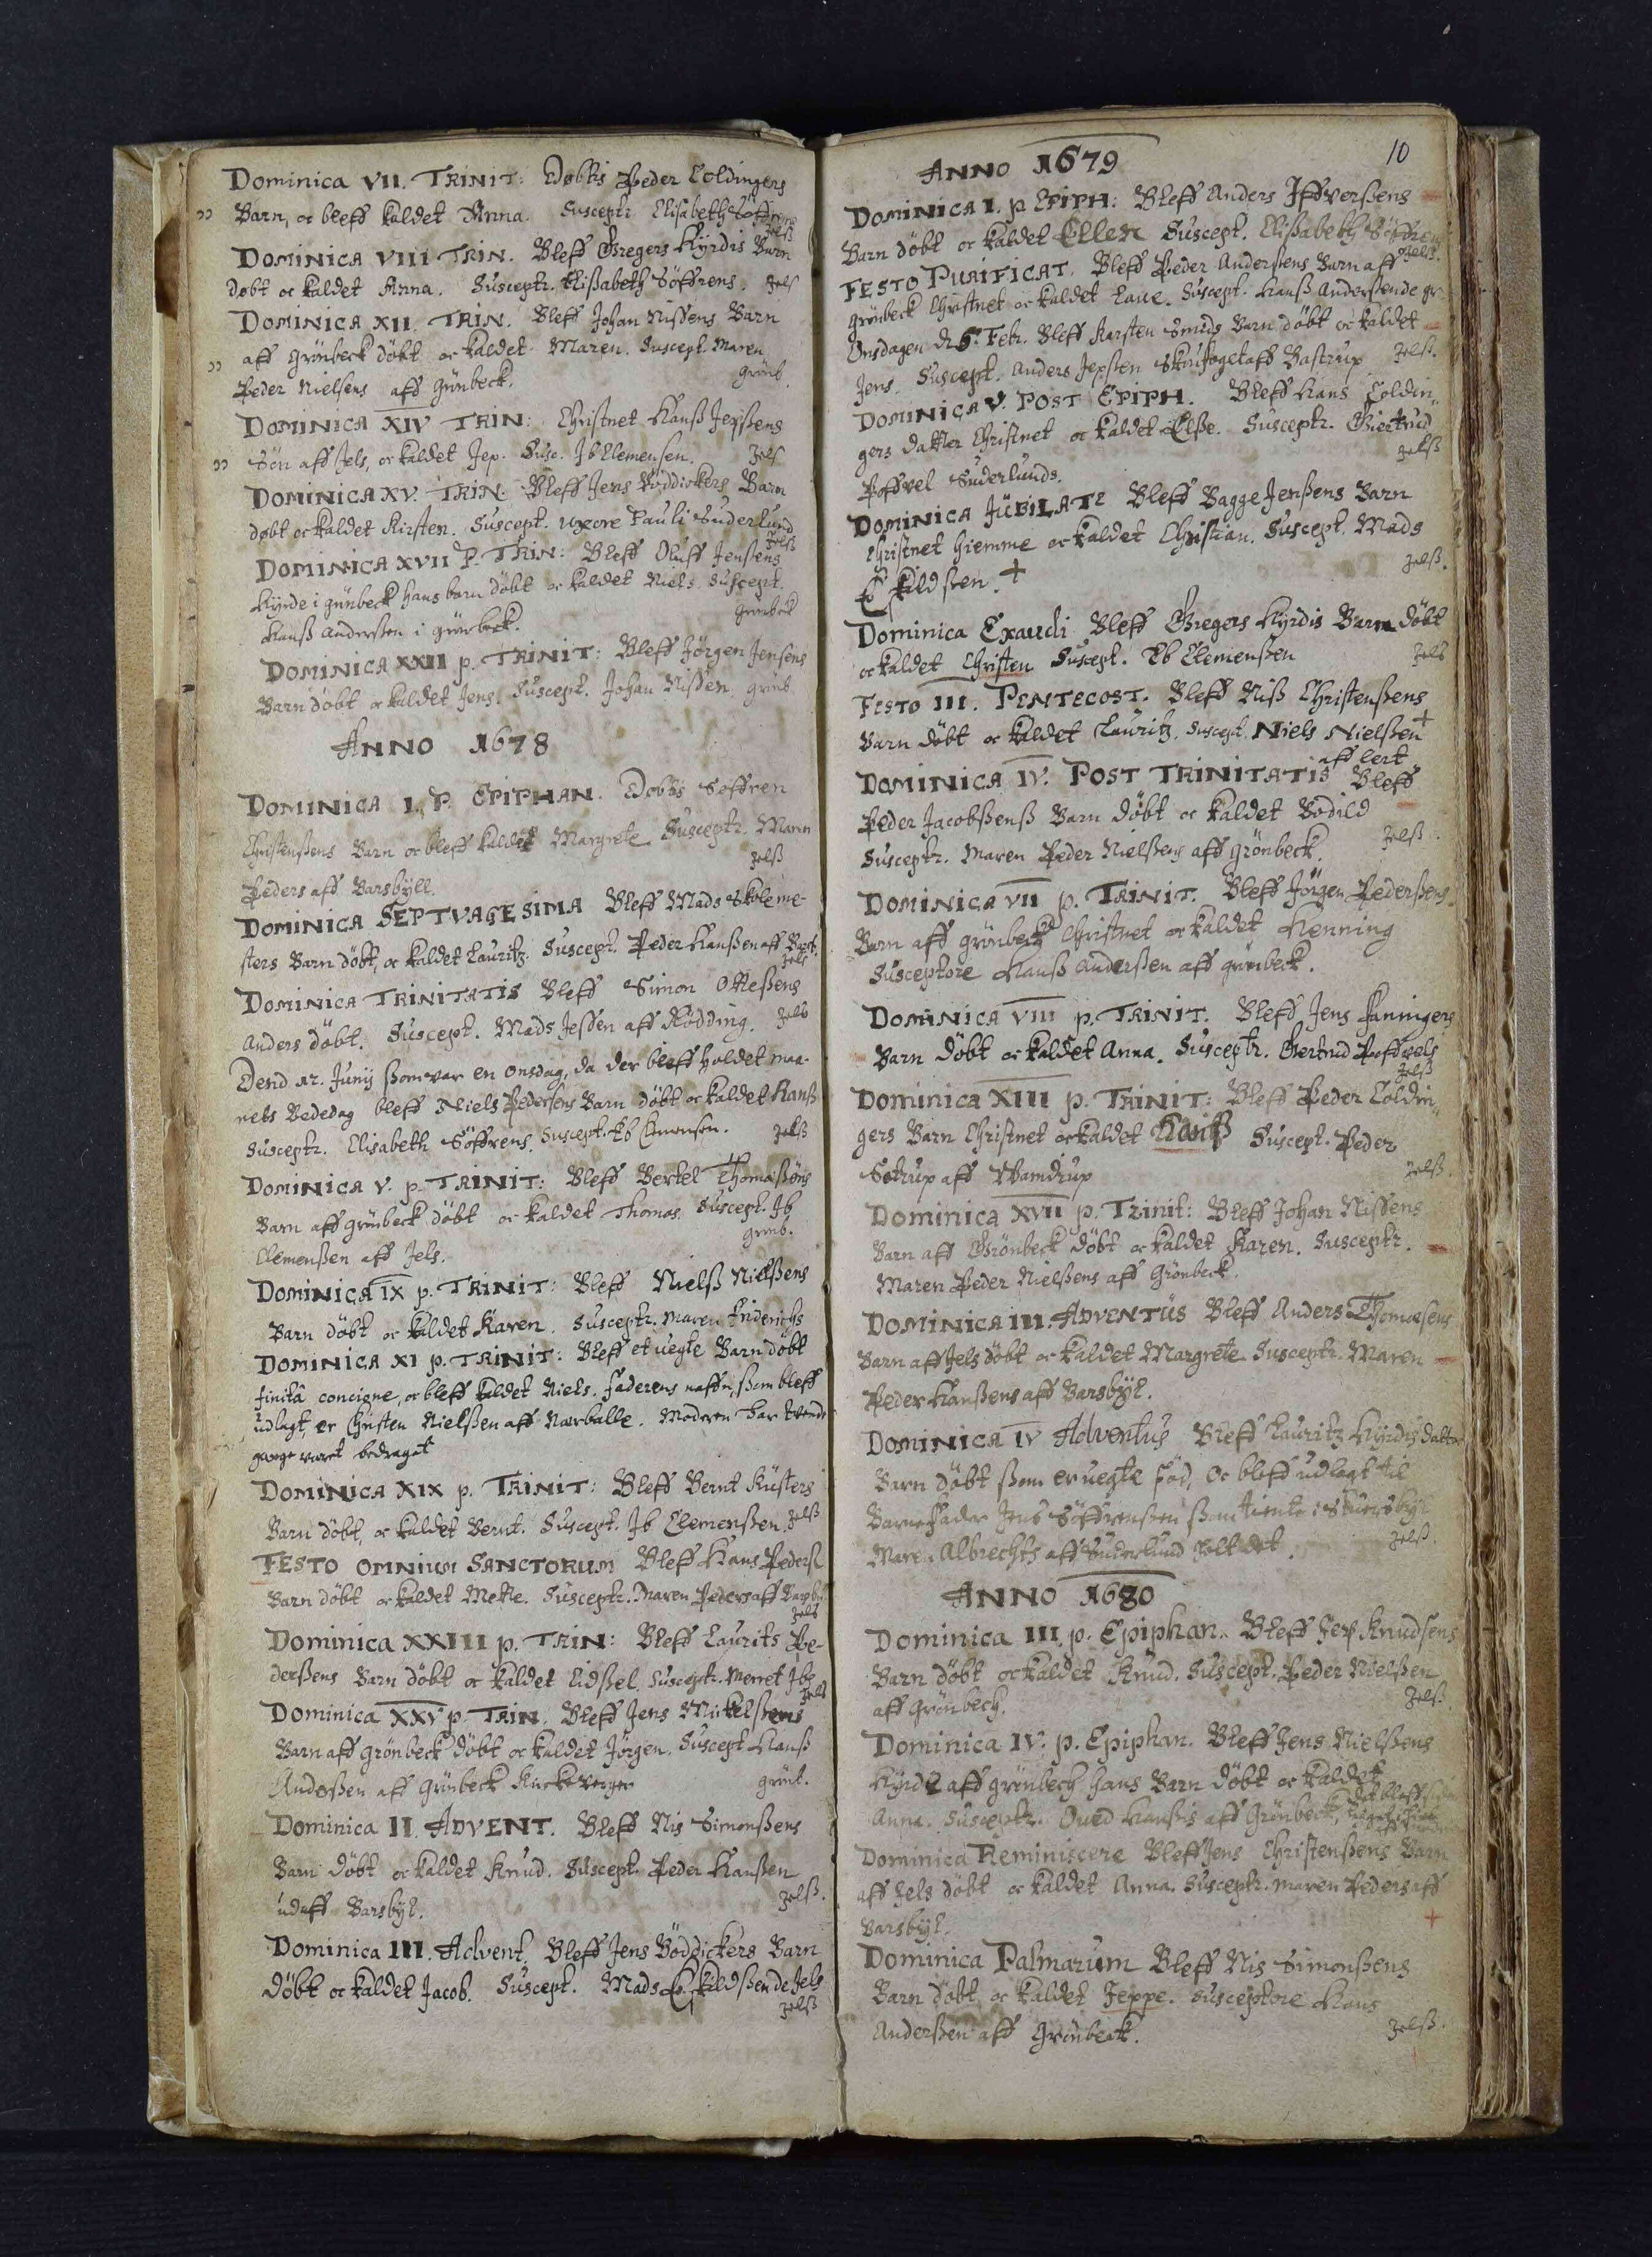Dominica VII TRINIT. Døbtis Peder Coldingers
Barn, oc bleff kaldet Anna. Susceptr Elisabeth Søffre##
Jels
DOMINICA VIII TRIN. Bleff Gregers Hÿrdis Barn
døbt oc kaldet Anna. Susceptr Elisabeth Søffrens. Jels
DOMINICA XII TRIN. Bleff Johan Nissens Barn
aff Grønbeck døbt oc kaldet Maren. Suscept. Maren
Peder Nielsens af Grønbeck.
Grønb
DOMINICA XIV TRIN: Christnet Hans Jepsens
Søn af Jels, oc kaldet Jep. Susc. Ib Clemensen. Jels
DOMINICA XV. TRIN. Bleff Jens Bøddickers Barn
døbt oc kaldet Kirsten. Suscept. Uxore Pauli Suderlund.
Jels
DOMINICA XVII P. TRIN: Bleff Oluf Jensens
Hÿrde i grønbeck hans barn døbt oc kaldet Niels Suscept.
Hans Andersen i grønbeck.
Grønbeck
DOMINICA XXII p. TRINIT: Bleff Jørgen Jensens
Barn døbt oc kaldet Jens. Suscept. Johan Nissen Grønb.
ANNO 1678
DOMINICA I P. EPIPHAN. Døbtis Søffren
Christensens Barn oc bleff kaldet Margrete. Susceptr. Maren
Peders aff Barsbyll.
Jels
DOMINICA SEPTVAGESIMA Bleff Mads Skoleme¬
sters Barn døbt, oc kaldet Lauritz. Suscept. Peder Hansen aff Barsb.
Jels
DOMINICA TRINITASTIS Bleff Simon Ottesens
Anders døbt. Suscept. Mads Jessen aff Rødding. Jels
Dend 12. Junij som var en onsdag, da der bleff holdet maa¬
nets Bededag bleff Niels Pedersens Barn døbt oc kaldet Hans
Suscept. Elisabeth Søffrens. Suscept. Ib Clemensen. Jels
DOMINICA V. p. TRINIT: Bleff Bertel Thomasøns
Barn aff grønbeck døbt oc kaldet Thomas. Suscept. Ib
Clemensen aff Jels.
Grmb.
DOMINICA IX p. TRINIT: Bleff Niels Nielsens
Barn døbt oc kaldet Karen. Susceptr. Maren Friderichs
DOMINICA XI p. TRINIT. Bleff et uegte Barn døbt
finita concione, oc bleff kaldet Niels. Faderens naffn, som bleff
udlagt, er Christen Nielsen aff Nærballe. Moderen har tvende
gange været bedraget
DOMINICA XIX p. TRINIT: Bleff Bernt Kusters
Barn døbt, oc kaldet Bernt. Suscept Ib Clemensen. Jels
FESTO OMNIUM SANCTORUM Bleff Hans Peders
Barn døbt oc kaldet Mette. Susceptr. Maren Peders aff Barsby
Jels
Dominica XXIII p. TRIN: Bleff Lauritz Pe¬
dersens Barn døbt oc kaldet Zidsel. Susceptr. Merret Ibs
Jels
Dominica XXV p. TRIN. Bleff Jens Mickelsens
Barn aff Grønbeck døbt oc kaldet Jørgen. Suscept Hans
Andersen aff Grønbeck kirkespgn
Grønb.
Dominica II ADVENT. Bleff Nis Simonsens
Barn døbt oc kaldet Knud. Suscept. Peder Hansen
udaff Barsbyl.
Jels.
Dominica III Advent Bleff Jens Bøddickers Barn
døbt oc kaldet Jacob. Suscept. Mads Eskildsen de Jels.
Jels.
10
ANNO 1679
DOMINICA I p. EPIPH: Bleff Anders Iffersens
Barn døbt oc kaldet Ellen Suscept. Elisabeth Søffrens.
Jels
FESTO PURIFICAT. Bleff Peder Andersens Barn aff
Grønbeck christnet oc kaldet Laue. Suscept. Hans Andersen de Gr
Onsdagen d 5 Febr. Bleff Karsten Smids Barn døbt oc kaldet
Jens. Suscept. Anders Jepsen Skoufoget aff Bastrup. Jels.
DOMINIC V. POST EPIPH. Bleff Hans Coldin¬
gers Datter christnet oc kaldet Else. Susceptr. Giertrud
Poffvel Suderlunds.
Jels
DOMINICA JUBILATE Bleff Bagge Jensens Barn
christnet hiemme oc kaldet Christian. Suscept. Mads
Eskildsen.
Jels.
Dominica Exaudi Bleff Gregers Hyrdis Barn døbt
oc kaldet Christen Suscept. Ib Clemensen
Jels
FESTO III. PENTECOST. Bleff Niss Christensens
Barn døbt oc kaldet Lauritz. Suscept Niels Nielsen
aff Lert
DOMINICA IV. POST TRINITATIS Bleff
Peder Jacobssens Barn døbt oc kaldet Bodild
Susceptr. Maren Peder Nielsens aff Grønbeck. Jels.
DOMINICA VII p. TRINIT. Bleff Jørgen Pedersens
Barn aff Grønbeck christnet oc kaldet Henning
Susceptore Hans Andersen aff Grønbeck.
DOMONICA VIII p. TRINIT. Bleff Jens Fannigers
Barn døbt oc kaldet Anna. Susceptr. Gertrud Poffvels.
Jels
DOMONICA XIII p. TRINIT: Bleff Peder Coldin¬
gers Barn christnet oc kaldet Hans Suscept. Peder
Satrup aff Wamdrup.
Jels.
DOMINICA XVII p. TRINIt: Bleff Johan Nissens
Barn aff Grønbeck døbt oc kaldet Karen. Susceptr.
Maren Peder Nielsens aff Grønbeck.
DOMINICA III ASVENTUS Bleff Anders Thomesens
Barn aff Jels døbt oc kaldet Margrete. Susceptr. Maren
Peder Hansens aff Barsbyl.
DOMINICA IV Adventus Bleff Lauritz Hyrdis Datter
Barn døbt som en uegte fød, oc bleff udlagt til
Barnefader Jens Søffrensen som tiente i Stursbyl
Maren Albrechts aff Suderlund holt det.
Jels.
ANNO 1680
DOMINICA III p. Epiphan. Bleff Jep Knudsens
Barn døbt oc kaldet Knud. Suscept. Peder Nielsen
aff Grønbech.
Jels.
Dominica IV. P. Epiphan. Bleff Jens Nielsens
Hyrde aff Grønbeck hans Barn døbt oc kaldet
det bleff
Anna. Susceptr. Oved Hansis aff Grønbeck, ##get i j##
aff## ##
Dominica Reminiscere Bleff Jens Christensens Barn
aff Jels døbt oc kaldet Anna. Susceptr. Maren Peders aff
Barsbyl.
Dominica Palmarum Bleff Nis Simonsens
Barn døbt oc kaldet Jeppe. Susceptore Hans
Andersen aff Grønbeck.
Jels.